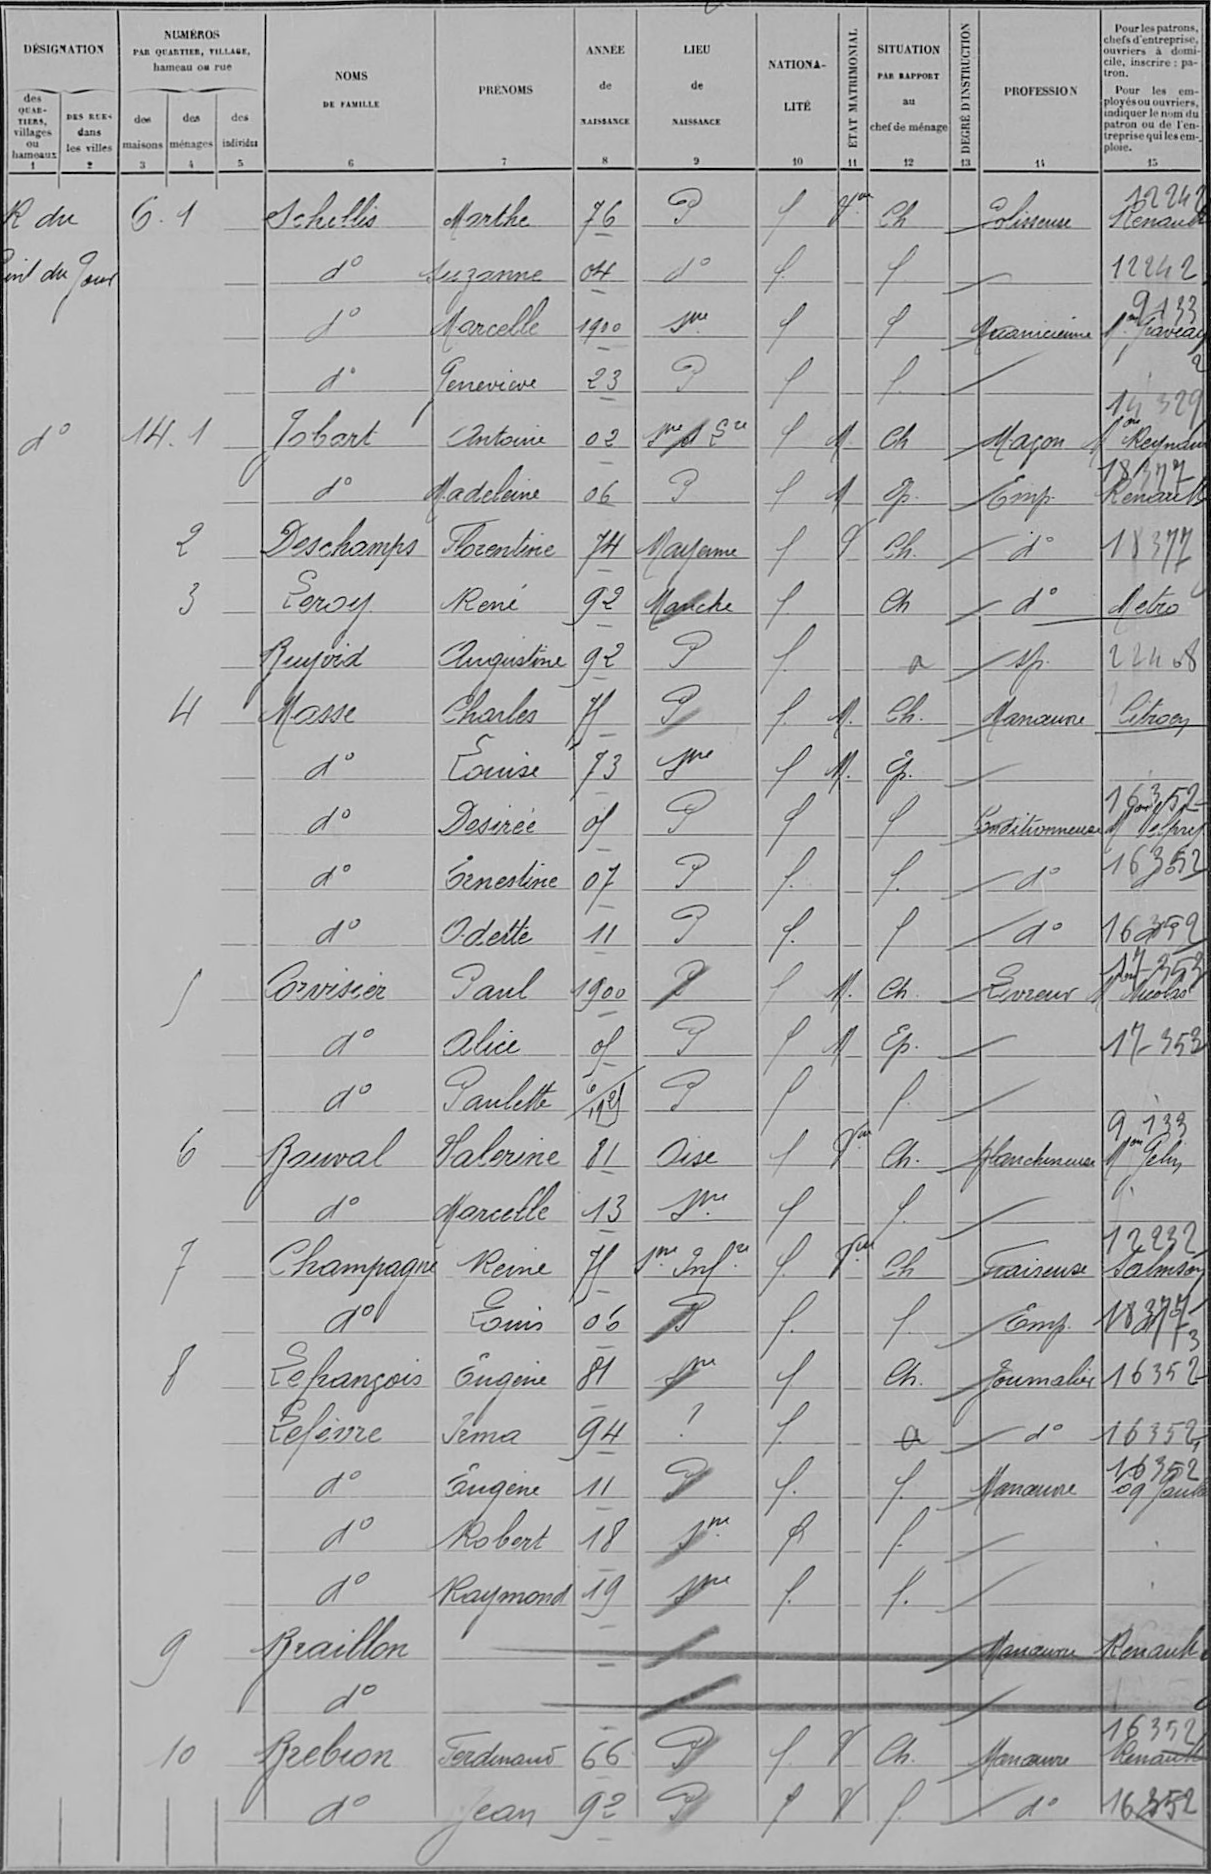Schillis/Marthe/76/P/f/V/Ch/¤/Polisseuse/Renault ?12242
d°/Suzanne/04/d°/f/¤/f/¤/¤/12242
d°/Marcelle/1900/Sne/f/¤/f/¤/Mecanicienne/Mon Graveau?9133
d°/Genevieve/23/P/f/¤/f/¤/¤/¤
Jobart/Antoine/02/Sne et Lre/f/M/ch/¤/Maçon/Mon Regnaud?14329
d°/Madeleine/06/P/f/M/Ep/¤/Emp/Renault?18377
Deschamps/Florentine/74/Mayenne/f/V/Ch/¤/d°/18377
Leroy/René/92/Manche/f/¤/Ch/¤/d°/Metro
Jujid/Augustine/92/P/f/¤/a/¤/sp/22408
Masse/Charles/75/P/f/M/Ch /¤/Manoeuvre/Citroen
d°/Louise/73/Sne/f/M /Ep /¤/¤/¤
d°/Desirée/05/P/f/¤/f/¤/conditionnement/Mon Delpry?16352
d°/Ernestine/07/P/f/¤/f/¤/d°/16352
d°/Odette/11/P/f /¤/f/¤/d°/16052
Corvisier/Paul/1900/P/f/M/Ch/¤/Livreur/Mon Nicolas?17353
d°/Alice/05/P/f/M/Ep /¤/¤/17353
d°/Paulette/1921/P/f/¤/f/¤/¤/¤
Bauval/Valerine/81/Oise/f/V/Ch /¤/blanchisseuse/Mon Gelin?9133
d°/Marcelle/13/Sne/f/¤/f/¤/¤/¤
Champagne/Reine/75/Sne Inf/f/V/Ch/¤/Fraiseuse/Salmson?12232
d°/Louis/06/P/f/¤/f/¤/Emp/18377
Lefrançois/Eugénie/81/Sne/f/¤/Ch /¤/journalier/16352
Lefevre/Irma/94/¤/f/¤/a/¤/d°/16352
d°/Eugene/11/P/f/¤/f/¤/Manoeuvre/oq auto?16352
d°/Robert/18/Sne/f/¤/f/¤/¤/¤
d°/Raymond/19/Sne/f/¤/f/¤/¤/¤
Braillon/¤/¤/¤/¤/¤/¤/¤/Manoeuvre/Renault
d°/¤/¤/¤/¤/¤/¤/¤/¤/¤
Rebion/Ferdinand/66/P/f/V/Ch /¤/Manoeuvre/Renault?16352
d°/Jean/92/P/f/V/f/¤/d°/16352
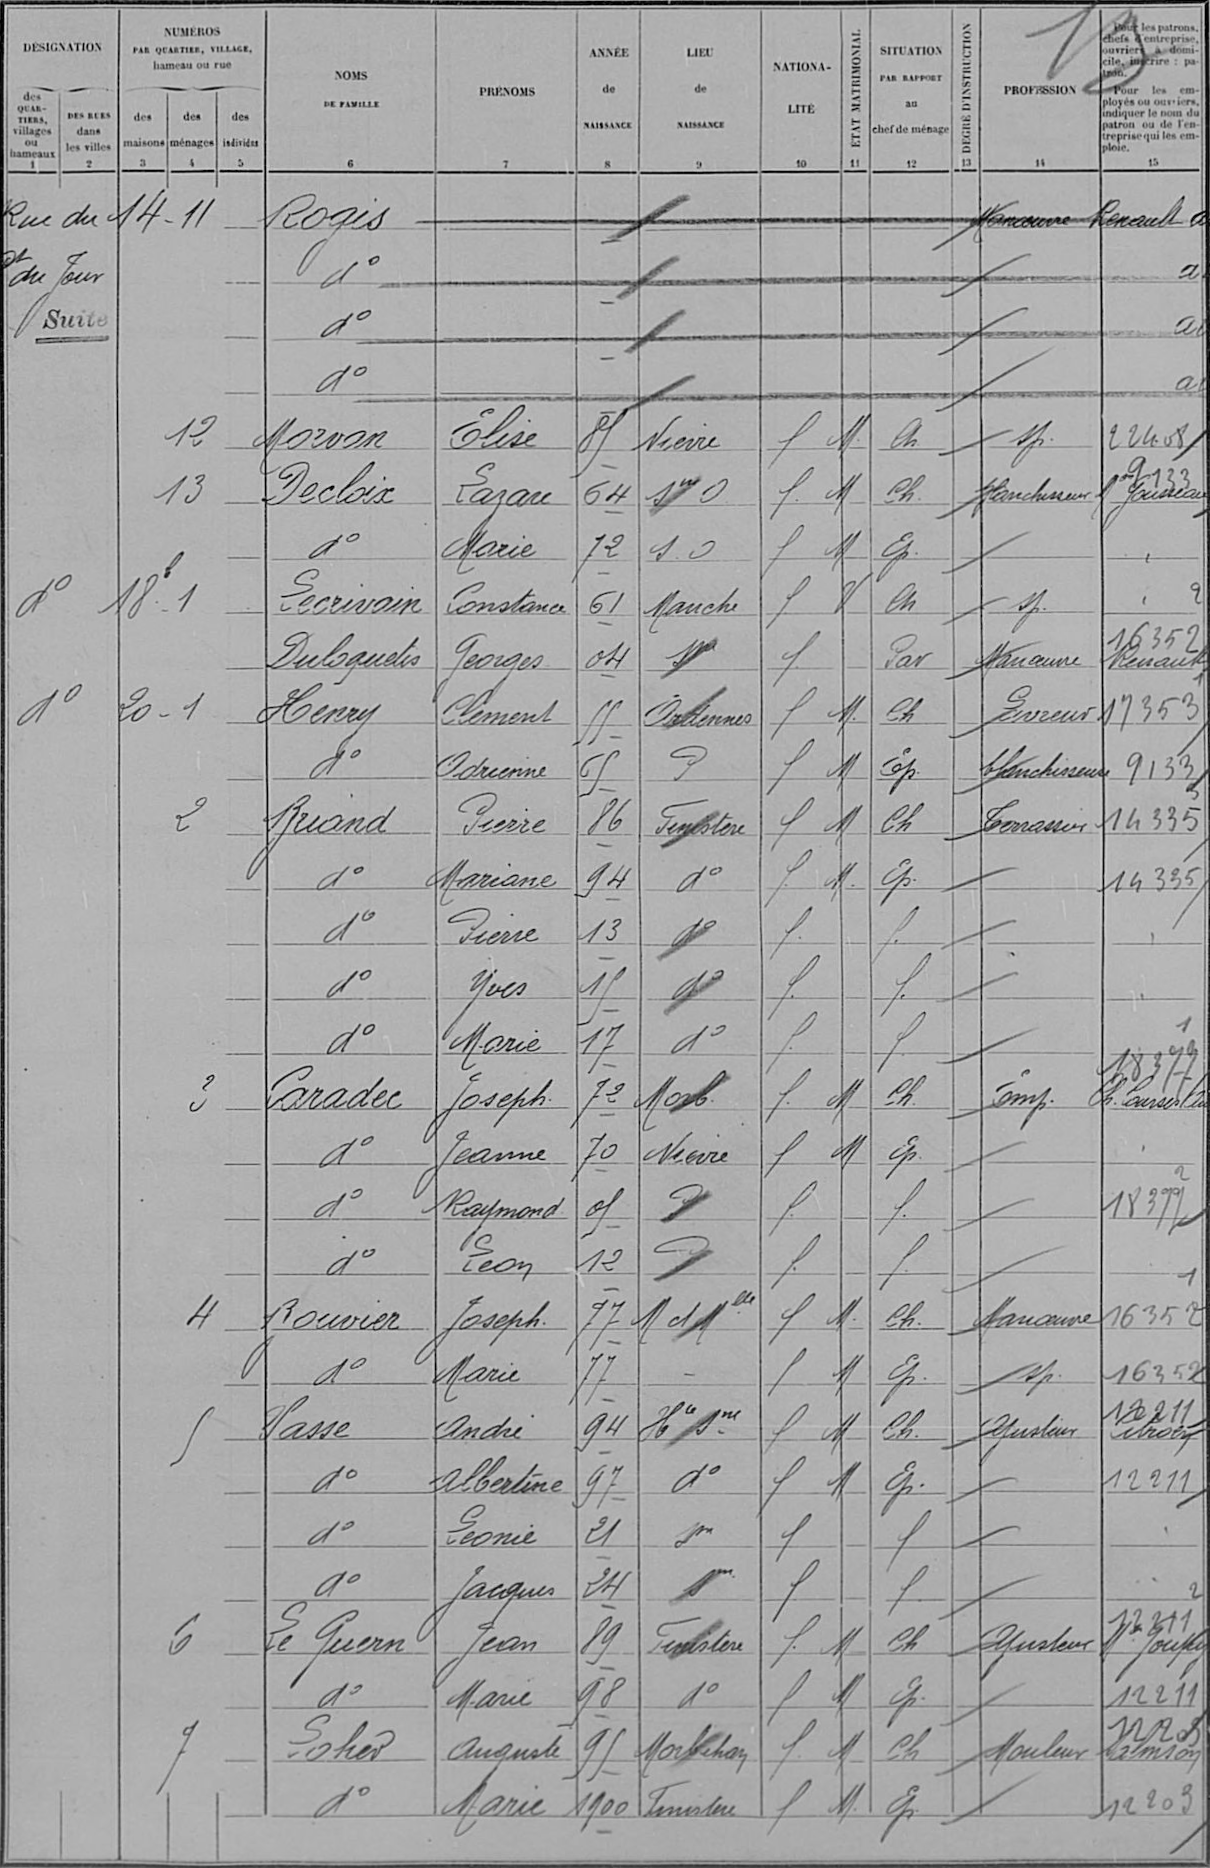Rogis/¤/¤/¤/¤/¤/¤/¤/Manoeuvre/Renault a
d°/¤/¤/¤/¤/¤/¤/¤/¤/a
d°/¤/¤/¤/¤/¤/¤/¤/¤/a
d°/¤/¤/¤/¤/¤/¤/¤/¤/a
Morvan/Elise/81/Nièvre/f/M/Ch/¤/sp/22408
Decloix/Lazare/64/Sn O/f/M/ch /¤/blanchisseuse/Mon Jousseau?9133
d°/Marie/72/SO/f/M/Ep/¤/¤/¤
Lecrivain/Constance/61/Manche/f/V/Ch/¤/sp /2
Duloquetis/Georges/04/Sne/f/¤/Par/¤/Manoeuvre/Renault?16352
Henry/Clement/55/Ardennes/f/M/Ch/¤/Livreur/17353
d°/Adrienne/65/P/f/M/Ep/¤/blanchisseuse/9133
Juand/Pierre/86/Finistere/f/M/Ch/¤/Terrassier/14335
d°/Mariane/94/d°/f/M/Ep/¤/¤/14335
d°/Pierre/13/d°/f/¤/f/¤/¤/¤
d°/Yves/15/d°/f/¤/f/¤/¤/¤
d°/Marie/17/d°/f/¤/f/¤/¤/¤
Caradec/Joseph/72/Nord/f/M/Ch /¤/Emp/Ch Courses?18377
d°/Jeanne/70/Nièvre/f/M/Ep/¤/¤/2
d°/Raymond/05/P/f/¤/f/¤/¤/18399
d°/Leon/12/P/f/¤/f/¤/¤/1
Rouvier/Joseph/77/M et Mlle/f/M/Ch /¤/Manoeuvre/16352
d°/Marie/77/¤/f/M/Ep /¤/sp/16352
Vasse/André/94/Hte Sne/f/M/Ch /¤/Ajusteur/Citroen?12211
d°/Albertine/97/d°/f/M/Ep /¤/¤/12211
d°/Léonie/21/d°/f/¤/f/¤/¤/¤
d°/Jacques/24/d°/f/¤/f/¤/¤/2
Le Guern/Jean/89/Finistère/f/M/Ch/¤/ajusteur/Mon Gouply?12211
d°/Marie/98/d°/f/M/Ep /¤/¤/12211
Loher/Auguste/95/Morbihan/f/M/Ch/¤/Mouleur/Salmson?12208
d°/Marie/1900/Finistère/f/M/Ep/¤/¤/12203